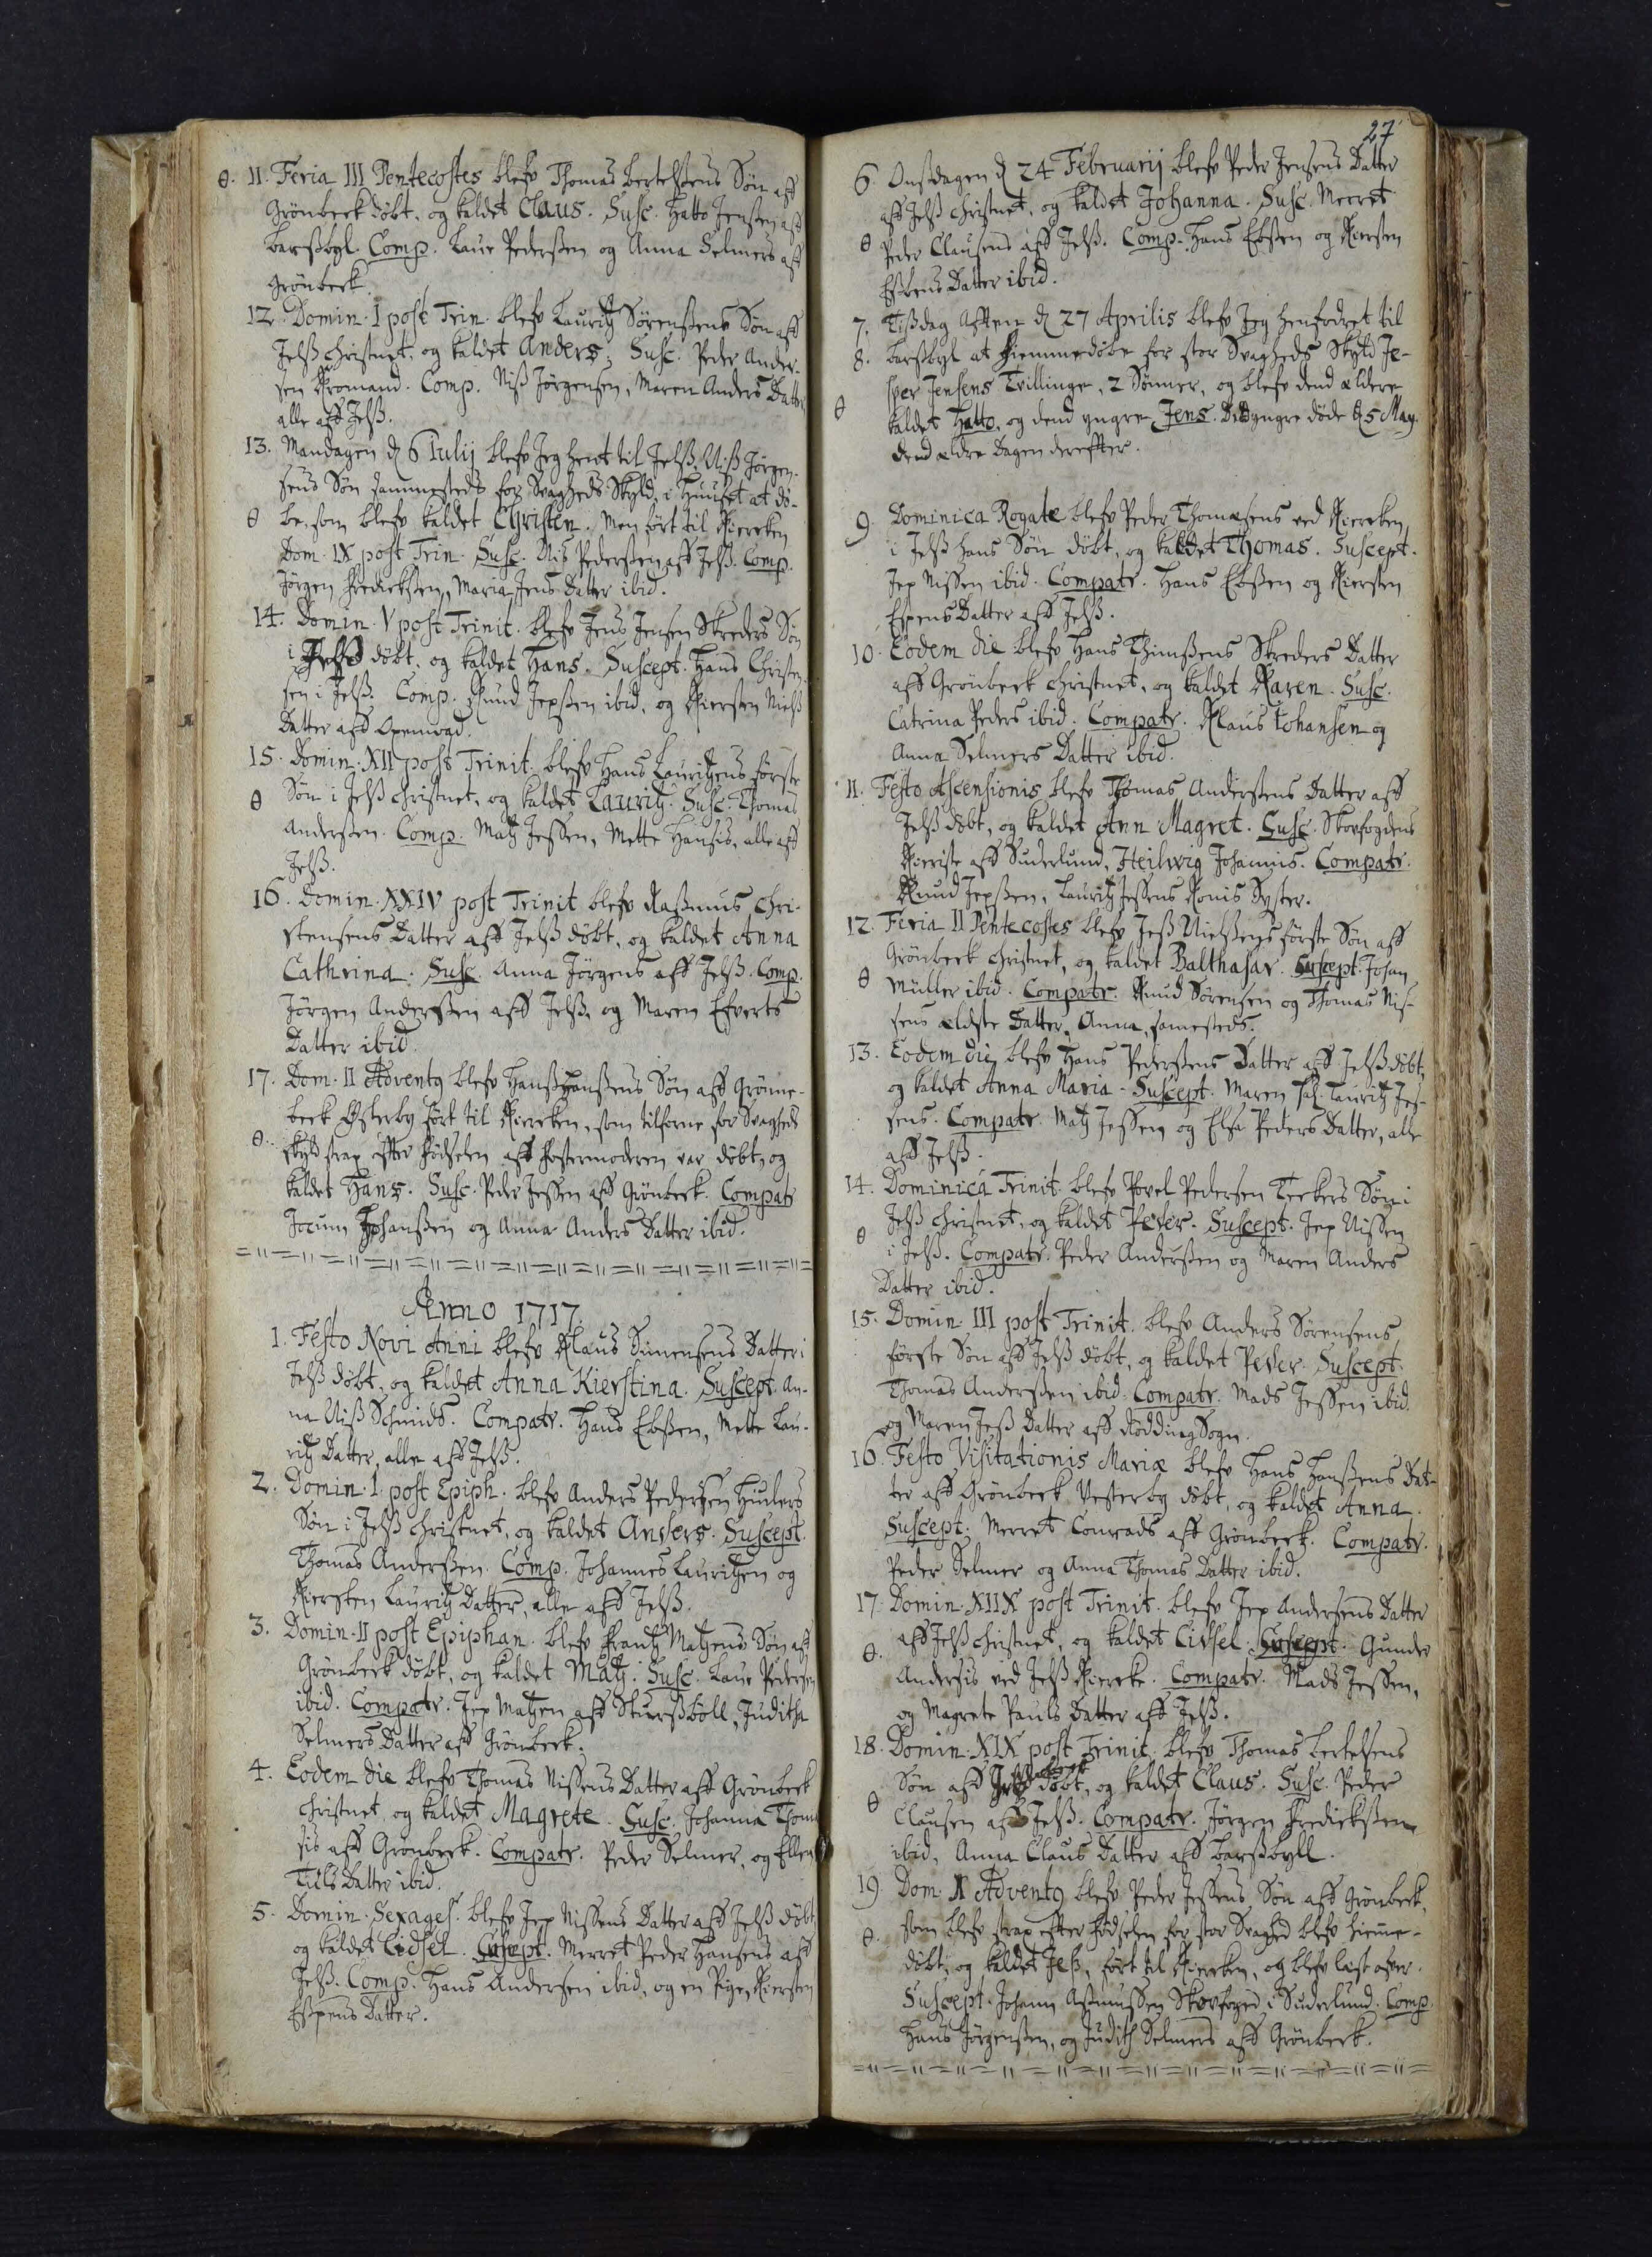11. Feria III Pentecostes blefv Thomas Bertelsens Søn aff
Grønbeck døbt, og kaldet Claus . Susc. Hatto Jensen aff
Barssbyl. Comp. Laue Pederssen og Anna Selmers aff
Grønbeck
12. Domin. I post Trin. blefv Lauritz Sørenssens Søn aff
Jelss christnet, og kaldet Anders, Susc. Peder Ander¬
sen Kromand. Comp. Niss Jørgensen, Maren Anders Datter,
alle aff Jelss.
13. Mandagen d 6 Iulij blef Jeg hent til Jelss, Niss Jørgen¬
sens Søn sammesteds for Svagheds Skyld, i Huuset at dø¬
be, som blef kaldet Christen . men ført til Kiercken
Dom. IX post Trin. Susc. Nis Pedersen aff Jelss. Comp.
Jørgen Fredicksen, Maria Jens datter ibid.
14. Domin. V post Trinit. blefv Jens Jensen Skreders Søn
døbt, og kaldet Hans. Suscept. Hans Christen¬
sen i Jelss. Comp. Knud Jepssen ibid, og Kiersten Nielss
Datter aff Oxemvad.
15. Domin. XII post Trinit. blefv Hans Lauritzens første
Søn i Jelss christnet, og kaldet Lauritz . Susc. Thomas
Andersen. Comp. Matz Jessen, Mette Hansis, alle aff
Jelss.
16. Domin. XXIV post Trinit blefv Rassmus Chri¬
stensens Datter aff Jelss døbt, og kaldet Anna
Cathrina. Susc. Anna Jørgens aff Jelss. Comp.
Jørgen Anderssen aff Jelss og Maren Efverts
Datter ibid
17. Dom. II Adventq blefv Hans Hanssens Søn aff Grønne¬
beck Østerby ført til Kiercken, som tilforne for Svagheds
skyld strax efter Fødslen aff Fostermoderen var døbt, og
kaldet Hans. Susc. Peder Jessen aff Grønbeck. Compatr
Jocum Johanssen og Anna Anders Datter ibid.
Anno 1717.
1. Festo Novi Anni blefv Klans Simensens Datter i
Jelss døbt, og kaldet Anna Kierstina. Suscept. An¬
na Niss Schmids. Compatr. Hans Ebssen, Mette Lau¬
ritz Datter, alle aff Jelss.
2. Domin. I post Epiph. blefv Anders Pedersen Hiulers
Søn i Jelss christnet, og kaldet Anders. Suscept.
Thomas Anderssen. Comp. Johannes Lauritzen og
Kiersten Lauritz Datter, alle aff Jelss.
3. Domin. II post Epiphan. blef Frantz Matzens Søn aff
Grønbeck døbt, og kaldet Matz. Susc. Laue Pedersen
ibid. Compatr. Jep Matzen aff Stursbøll, Judith
Selmers Datter aff Grønbeck.
4. Eodem die blefv Thomas Nissens Datter aff Grønbeck
christnet og kaldet Magrete. Susc. Johanna Thomis
sis aff Grønbeck. Compatr. Peder Selmers, og Ellen
Tiils Datter ibid.
5. Domin. Sexages. blefv Jep Nissens Datter aff Jelss døbt
og kaldet Cidsel. Cuscept. Merret Peder Clausens aff
Jelss. Comp. Hans Andersen ibid, og en Pige Kiersten
Essbens Datter.
27
6. Onsdagen d 24 Februarij blefv Peder Jensens Datter
aff Jelss christnet, og kaldet Johanna. Susc. Merret
Peder Clausens aff Jelss. Comp. Hans Ebssen og Kiersten
Essbens Datter ibid.
7. Tissdag Aften d 27 Aprilis blefv Jeg henfodret til
8. Barssbyl at hiemmedøbe for stor Svagheds Skyld Je¬
sper Jensens Tvillinge, 2 Sønner, og blefv dend aldere
kaldet Hatto, og dend yngre Jens. Dend yngre døde d 5 Maij.
dend ældre Dagen dereffter.
9. Dominica Rogate blefv Peder Thomasens ved Kiercken
i Jelss hans Søn døbt, og kaldet Thomas. Suscept.
Jep Nissen ibid. Compatr. Hans Ebssen og Kiersten
Espens Datter aff Jelss.
10. Eodem die blefv Hans Thimssens Skreders Datter
aff Grønbeck christnet, og kaldet Karen. Susc.
Catrina Peders ibid. Compatr. Klaus Iohansen og
Anna Selmers Datter ibid.
11. Festo Ascensionis blefv Thomas Anderssens Datter aff
Jelss døbt, og kaldet Ann Magret. Susc. Skovfogdens
Kieriste aff Suderlund, Heilwig Johannis. Compatr.
Knud Jepssen, Lauritz Jessens Konis Syster.
12. Feria II Pentecostes blefv Jess Nielssens første Søn aff
Grønbeck christnet, og kaldet Balthasar . Suscept. Johan
Muller ibid. Compatr. Knud Sørensen og Thomas Nis¬
sens aldste Datter, Anna, samesteds.
13. Eodem die blefv Hans Pederssens Datter aff Jelss døbt,
og kaldet Anna Maria. Suscept. Maren sal. Lauritz Jes¬
sens. Compatr. Matz Jessen og Elsa Peders Datter, alle
aff Jelss.
14. Dominica Trinit. blefv Povel Pedersen Teckers Søn i
Jelss christnet, og kaldet Peder . Suscept. Jep Nissen
i Jelss. Compatr. Peder Anderssen og Maren Anders
Datter ibid.
15. Domin. III post Trinit. blefv Anders Sørensens
første Søn aff Jelss døbt, og kaldet Peder. Suscept.
Thomas Anderssen ibid. Compatr. Mads Jessen ibid.
og Maren Jess Datter aff Rødding Sogn.
16. Festo Visitationis Maria blef Hans Hansens Dat¬
ter aff Grønbeck Vesterby døbt, og kaldet Anna.
Suscept. Merret Conrads aff Grønbeck. Compatr.
Peder Selmer og Anna Thomas Datter ibid.
17. Domin. XIIX post Trinit. blefv Jep Andersens Datter
aff Jelss christnet, og kaldet Cidsel. Suscept. Gunder
Andersis ved Jelss Kiercke. Compatr. Mads Jessen,
og Magrete Pauls Datter aff Jelss.
18. Domin. XIX post Trinit blefv Thomas Bertelsens
Søn aff ## døbt, og kaldet Claus . Susc. Peder
Clausen aff Jelss. Compatr. Jørgen Fredickssen
ibid. Anna Claus Datter aff Barssbyll.
19. Dom. I Advent blefv Peder Jessens Søn aff Grønbeck,
som blefv strax efter fødslen for stor Svaghed blefv hiemme¬
døbt, og kaldet Jess , ført til Kiercken, og blefv last ofver.
Suscept. Johann Assmussen Skovfoged i Suderlund. Comp.
Hans Jørgenssen, og Judith Selmers aff Grønbeck.